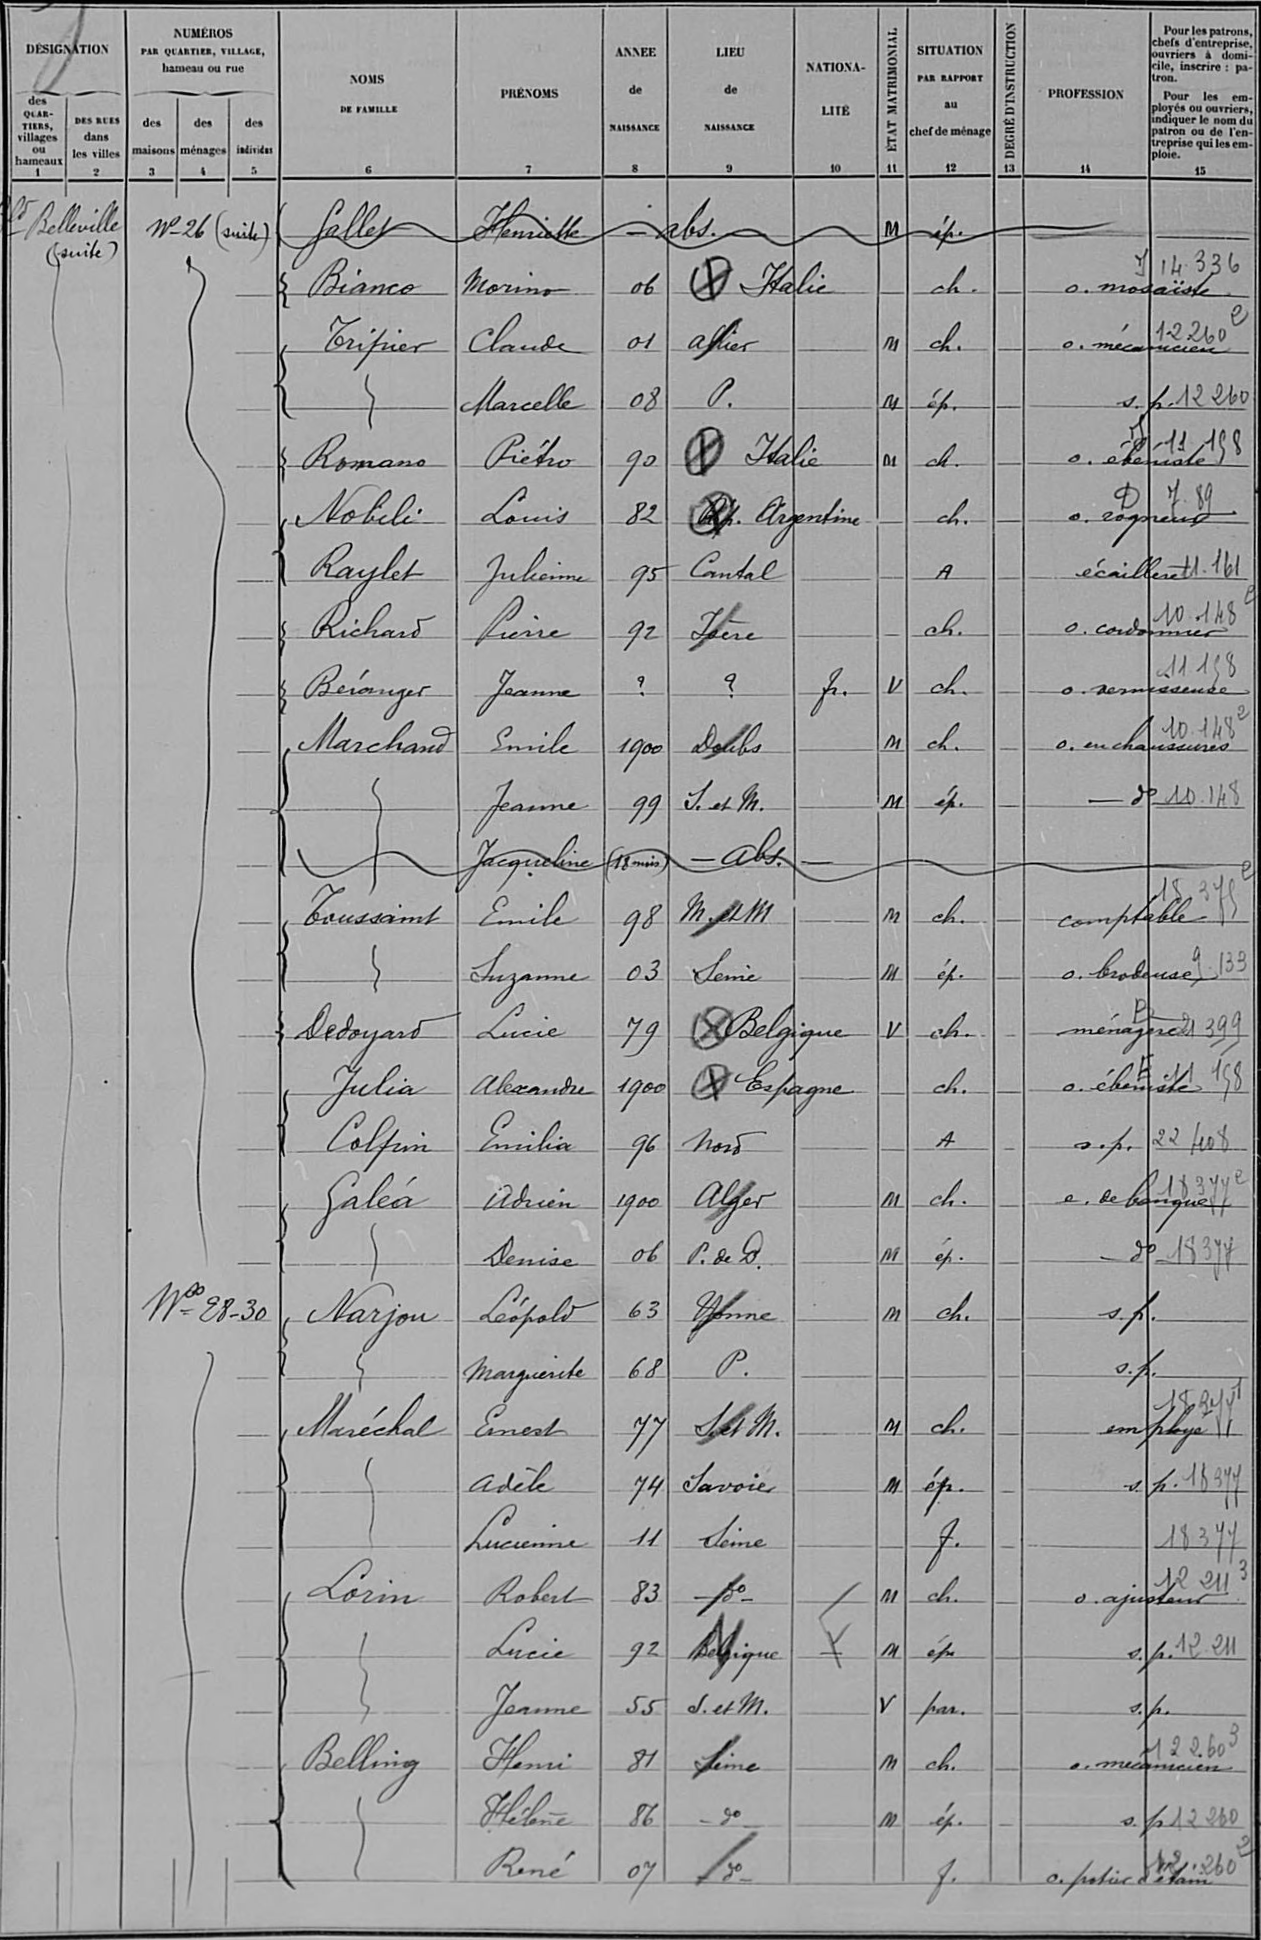Gallet/Henriette/¤/abs /¤/M/ép /¤/¤/¤/¤
Bianco/Morino/06/Italie/¤/¤/ch /¤/o mosaïste/¤
Tripier/Claude/01/Allier/¤/M/ch /¤/o mécanicien/¤
¤/Marcelle/08/P /¤/M/ép /¤/s p /12260
Romano/Piétro/90/Italie/M/ch /¤/o ébéniste/¤
Nobili/Louis/82/Rép Argentine/¤/¤/ch /¤/o rogneur/789
Raylet/Julienne/95/Cantal/¤/¤/A/¤/écaillère/11161
Richard/Pierre/92/Isère/¤/¤/ch /¤/o cordonnier/¤
Béranger/Jeanne/¤/¤/fr /V/ch /¤/o vernisseuse/¤
Marchand/Emile/1900/Doubs/¤/M/ch /¤/o en chaussures/¤
¤/Jeanne/99/S et M /¤/M/ép /¤/d°/10148
¤/Jacqueline/(18 mois)/abs /¤/¤/¤/¤/¤/¤/¤
Toussaint/Emile/98/M et M/¤/M/ch /¤/comptable/¤
¤/Suzanne/03/Seine/¤/M/ép /¤/o brodeuse/9133
Dedoyard/Lucie/79/Belgique/¤/V/ch /¤/ménagere/21399
Julia/Alexandre/1900/Espagne/¤/ch /¤/o ébéniste/11158
Colprin/Emilia/96/Nord/¤/¤/A/¤/s p /22408
Galéa/Adrien/1900/Alger/¤/M/ch /¤/e de banque/18377
¤/Denise/06/P de D /¤/M/ép /¤/d°/18377
Narjou/Léopold/63/Yonne/¤/M/ch /¤/s p /¤/¤
¤/Marguerite/68/P /¤/¤/¤/¤/s p /¤/¤
Maréchal/Ernest/77/S et M /¤/M/ch /¤/employé/¤
¤/Adèle/74/Savoie/¤/M/ép /¤/s p /18377
¤/Lucienne/11/Seine/¤/¤/f /¤/¤/183773
Lorin/Robert/83/d°/¤/M/ch /¤/o ajusteur/¤
¤/Lucie/92/Belgique/¤/M/ép/¤/s p /12211
¤/Jeanne/55/S et M /¤/V/par /¤/s p /¤/¤
Belling/Henri/81/Seine/¤/M/ch /¤/o mécanicien/¤
¤/Hélène/86/d°/¤/M/ép /¤/s p /122602
¤/René/07/d°/¤/¤/f /¤/o potier d'étain/¤
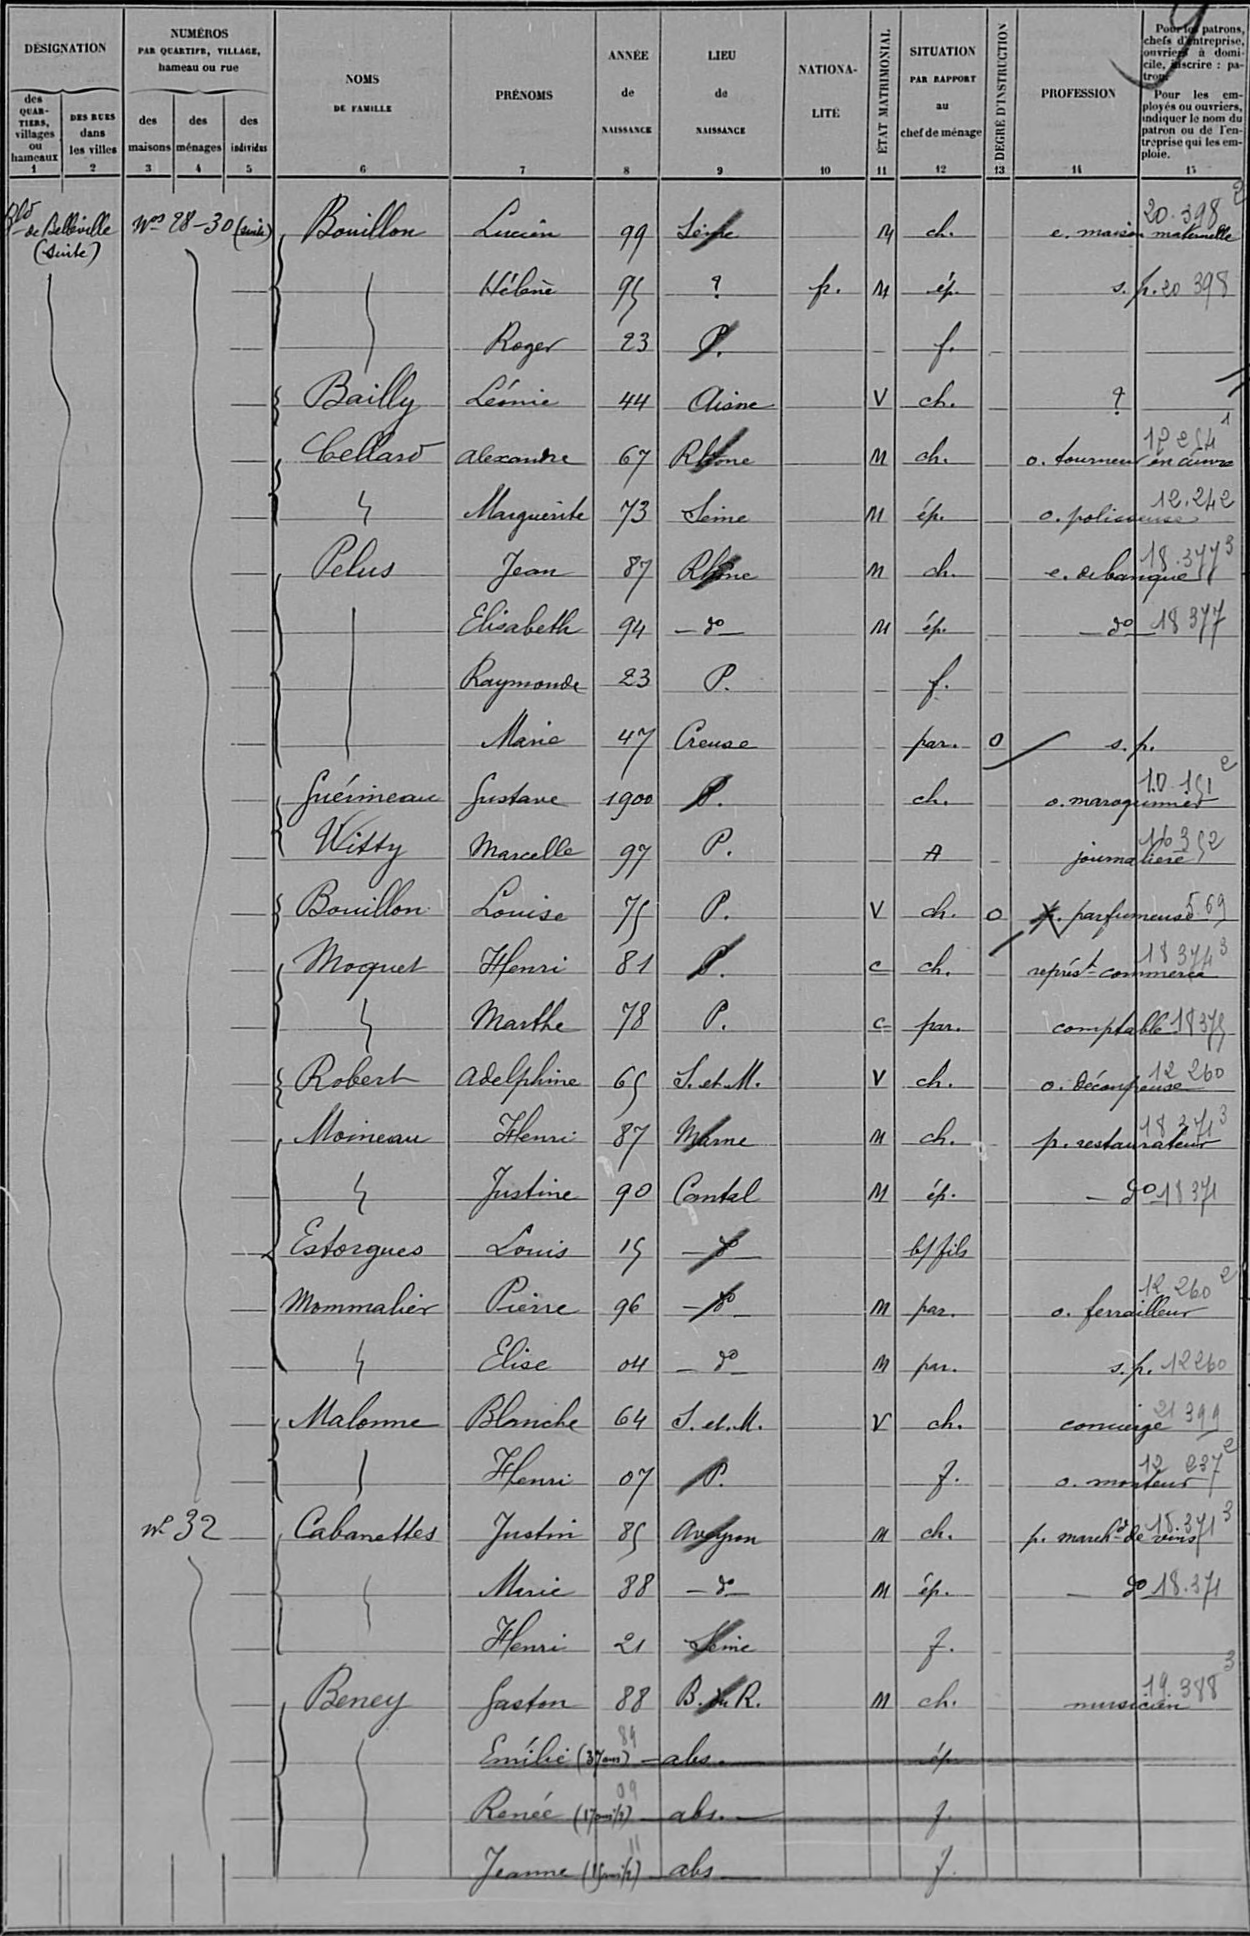Bouillon/Lucien/99/Seine/¤/M/ch /¤/e maison maternelle/¤
¤/Hélène/95/¤/fr /M/ép /¤/s pr /20398
¤/Roger/23/P /¤/¤/f /¤/¤/¤/¤
Bailly/Léonie/44/Aisne/¤/V/ch /¤/¤/¤/¤
Cellard/Alexandre/67/Rhone/¤/M/ch /¤/o tourneur en cuivre/¤
¤/Marguerite/73/Seine/¤/M/ép /¤/o polisseuse/¤
Pelus/Jean/87/Rhone/¤/M/ch /¤/e de banque/¤
¤/Elisabeth/94/d°/¤/M/ép /¤/d°/18377
¤/Raymonde/23/P /¤/¤/f /¤/¤/¤/¤
¤/Marie/47/Creuse/¤/¤/par /O/s pr /¤/¤
Guérmeau/Gustave/1900/P /¤/¤/ch /¤/o maroquinier/¤
Wissy/Marcelle/97/P /¤/¤/A/¤/journaliere/¤
Bouillon/Louise/75/P /¤/V/ch/o/parfumeuse/569
Moquet/Henri/81/P /¤/c/ch /¤/représt-commerce/¤
¤/Marthe/78/P /¤/c/par /¤/comptable/18375
Robert/Adelphine/65S et M /¤/V/ch /¤/o découpeuse/¤
Moineau/Henri/87/Marne/¤/M/ch /¤/p restaurateur/¤
¤/Justine/90/Cantal/¤/M/ép /¤/d°/18371
Estorgues/Louis/15/d°/¤/¤/b fils/¤/¤/¤/¤
Mommalier/Pierre/96/d°/¤/M/par /¤/o ferrailleur/¤
¤/Elise/04/d°/¤/M/par /¤/s p /12260
Malonne/Blanche/64/S et M /¤/V/ch /¤/concierge/21399
¤/Henri/07/P /¤/¤/f /¤/o monteur/122372
Cabanettes/Justin/85/Aveyron/¤/M/ch /¤/p marchd-de-vins/¤
¤/Marie/88/d°/¤/M/ép /¤/d°/18 371
¤/Henri/21/Seine/¤/¤/f /¤/¤/3/¤
Beney/Gaston/88/B du R /¤/M/ch /¤/musicien/¤
¤/Emilie (37 ans)/84/abs /¤/¤/ép/¤/¤/¤/¤
¤/Renée (17 ans 1 2)/09/abs /¤/¤/f /¤/¤/¤/¤
¤/Jeanne (15 ans 1 2)/11/abs/¤/¤/f /¤/¤/¤/¤
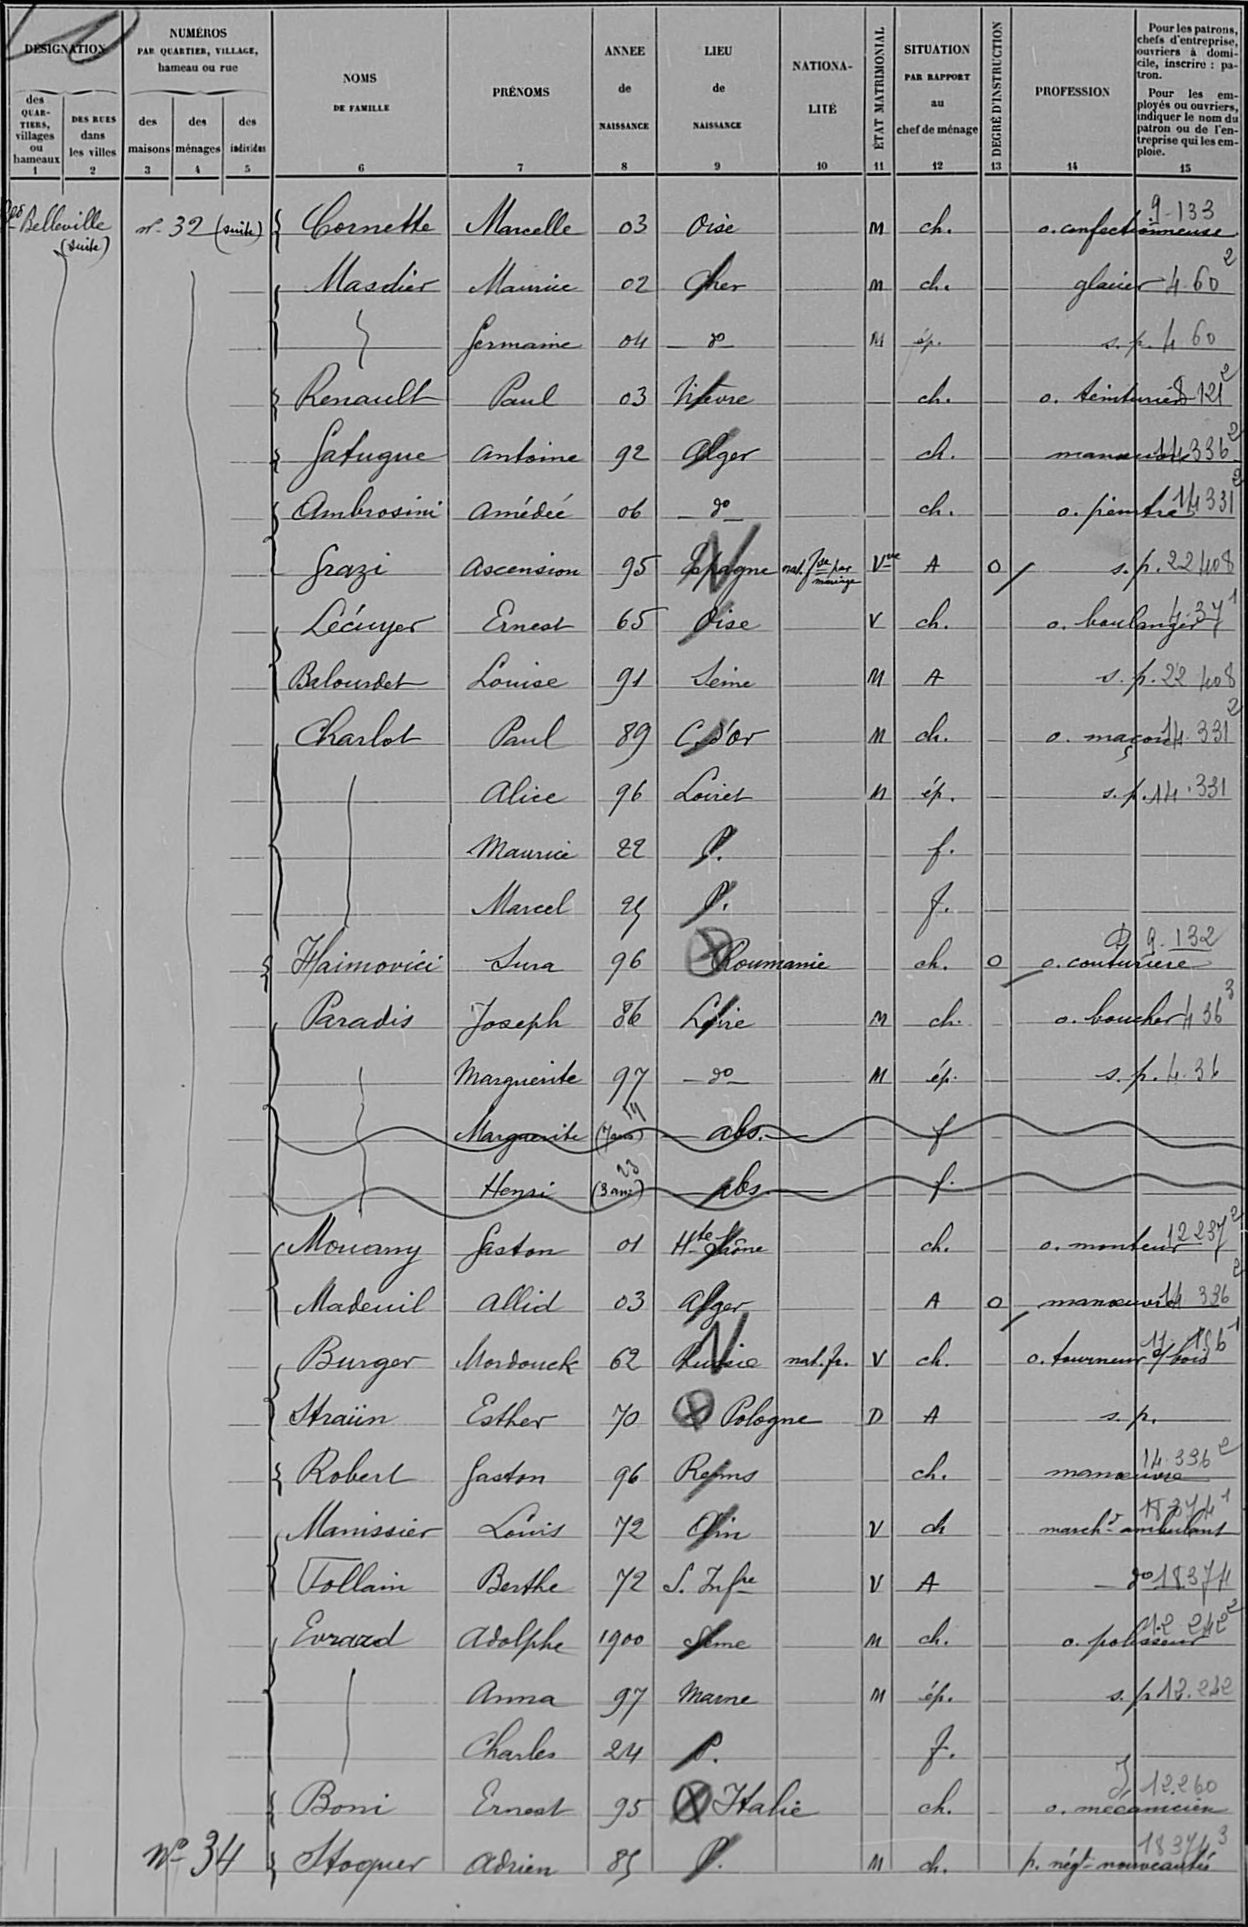Cornette/Marcelle/03/Oise/¤/M/ch /¤/o confectionneuse/¤
Masdier/Maurice/02/Cher/¤/M/ch /¤/glacier/460
¤/Germaine/04/d°/¤/M/ép /¤/s p /460
Renault/Paul/03/Nièvre/¤/¤/ch /¤/o teinturier/8121
Gahugue/Antoine/92/Alger/¤/¤/ch /¤/manoeu/143362
Ambrosini/Amédée/06/d°/¤/¤/ch /¤/o peintre/14331
Grazi/Ascension/95/Espagne/nat fr par/Vve/A/O/s p /22408
Lecuyer/Ernest/65/Oise/¤/V/ch /¤/o boulanger/37
Belourdet/Louise/91/Seine/¤/M/A/¤/s p /22408
Charlot/Paul/89/C d'Or/¤/M/ch /¤/o maçon/14331
¤/Alice/96/Loiret/¤/M/ép /¤/s p /14331
¤/Maurice/22/P /¤/¤/f /¤/¤/¤
¤/Marcel/25/P /¤/¤/f /¤/¤/¤
Haimovici/Sura/96/Roumanie/¤/¤/ch /0/o couturière/¤
Paradis/Joseph/86/Loire/¤/M/ch /¤/o boucher/436
¤/Marguerite/97/d°/¤/M/ép /¤/s p /436
¤/Marguerite/(7 ans)/abs /¤/¤/f/¤/¤/¤
¤/Henri/(3 ans)/abs /¤/¤/f /¤/¤/¤
Mouamy/Gaston/01/Hte Saône/¤/¤/ch /¤/o monteur/12237
Madeuil/Allid/03/Alger/¤/¤/A/O/manoeuvre/16336
Burger/Mordouck/62/Russie/nat fr /V/ch /¤/o tourneur s: bois/¤
Straün/Esther/70/Pologne/¤/D/A/¤/s p /¤
Robert/Gaston/96/Reims/¤/¤/ch /¤/manoeuvre/¤
Manissier/Louis/72/Ain/¤/V/ch/¤/marchd ambulant/¤
Vollain/Berthe/72/S Infre/¤/V/A/¤/d°/18374
Evrard/Adolphe/1900/Seine/¤/M/ch /¤/o polisseur/¤
¤/Anna/97/Maine/¤/M/ép /¤/s p /12342
¤/Charles/2:4/P /¤/¤/f /¤/¤/¤
Boni/Ernest/95/Italie/¤/¤/ch /¤/o mécanicien/¤
Hoquer/Adrien/85/P /¤/M/ch /¤/p négt-nouveautés/¤